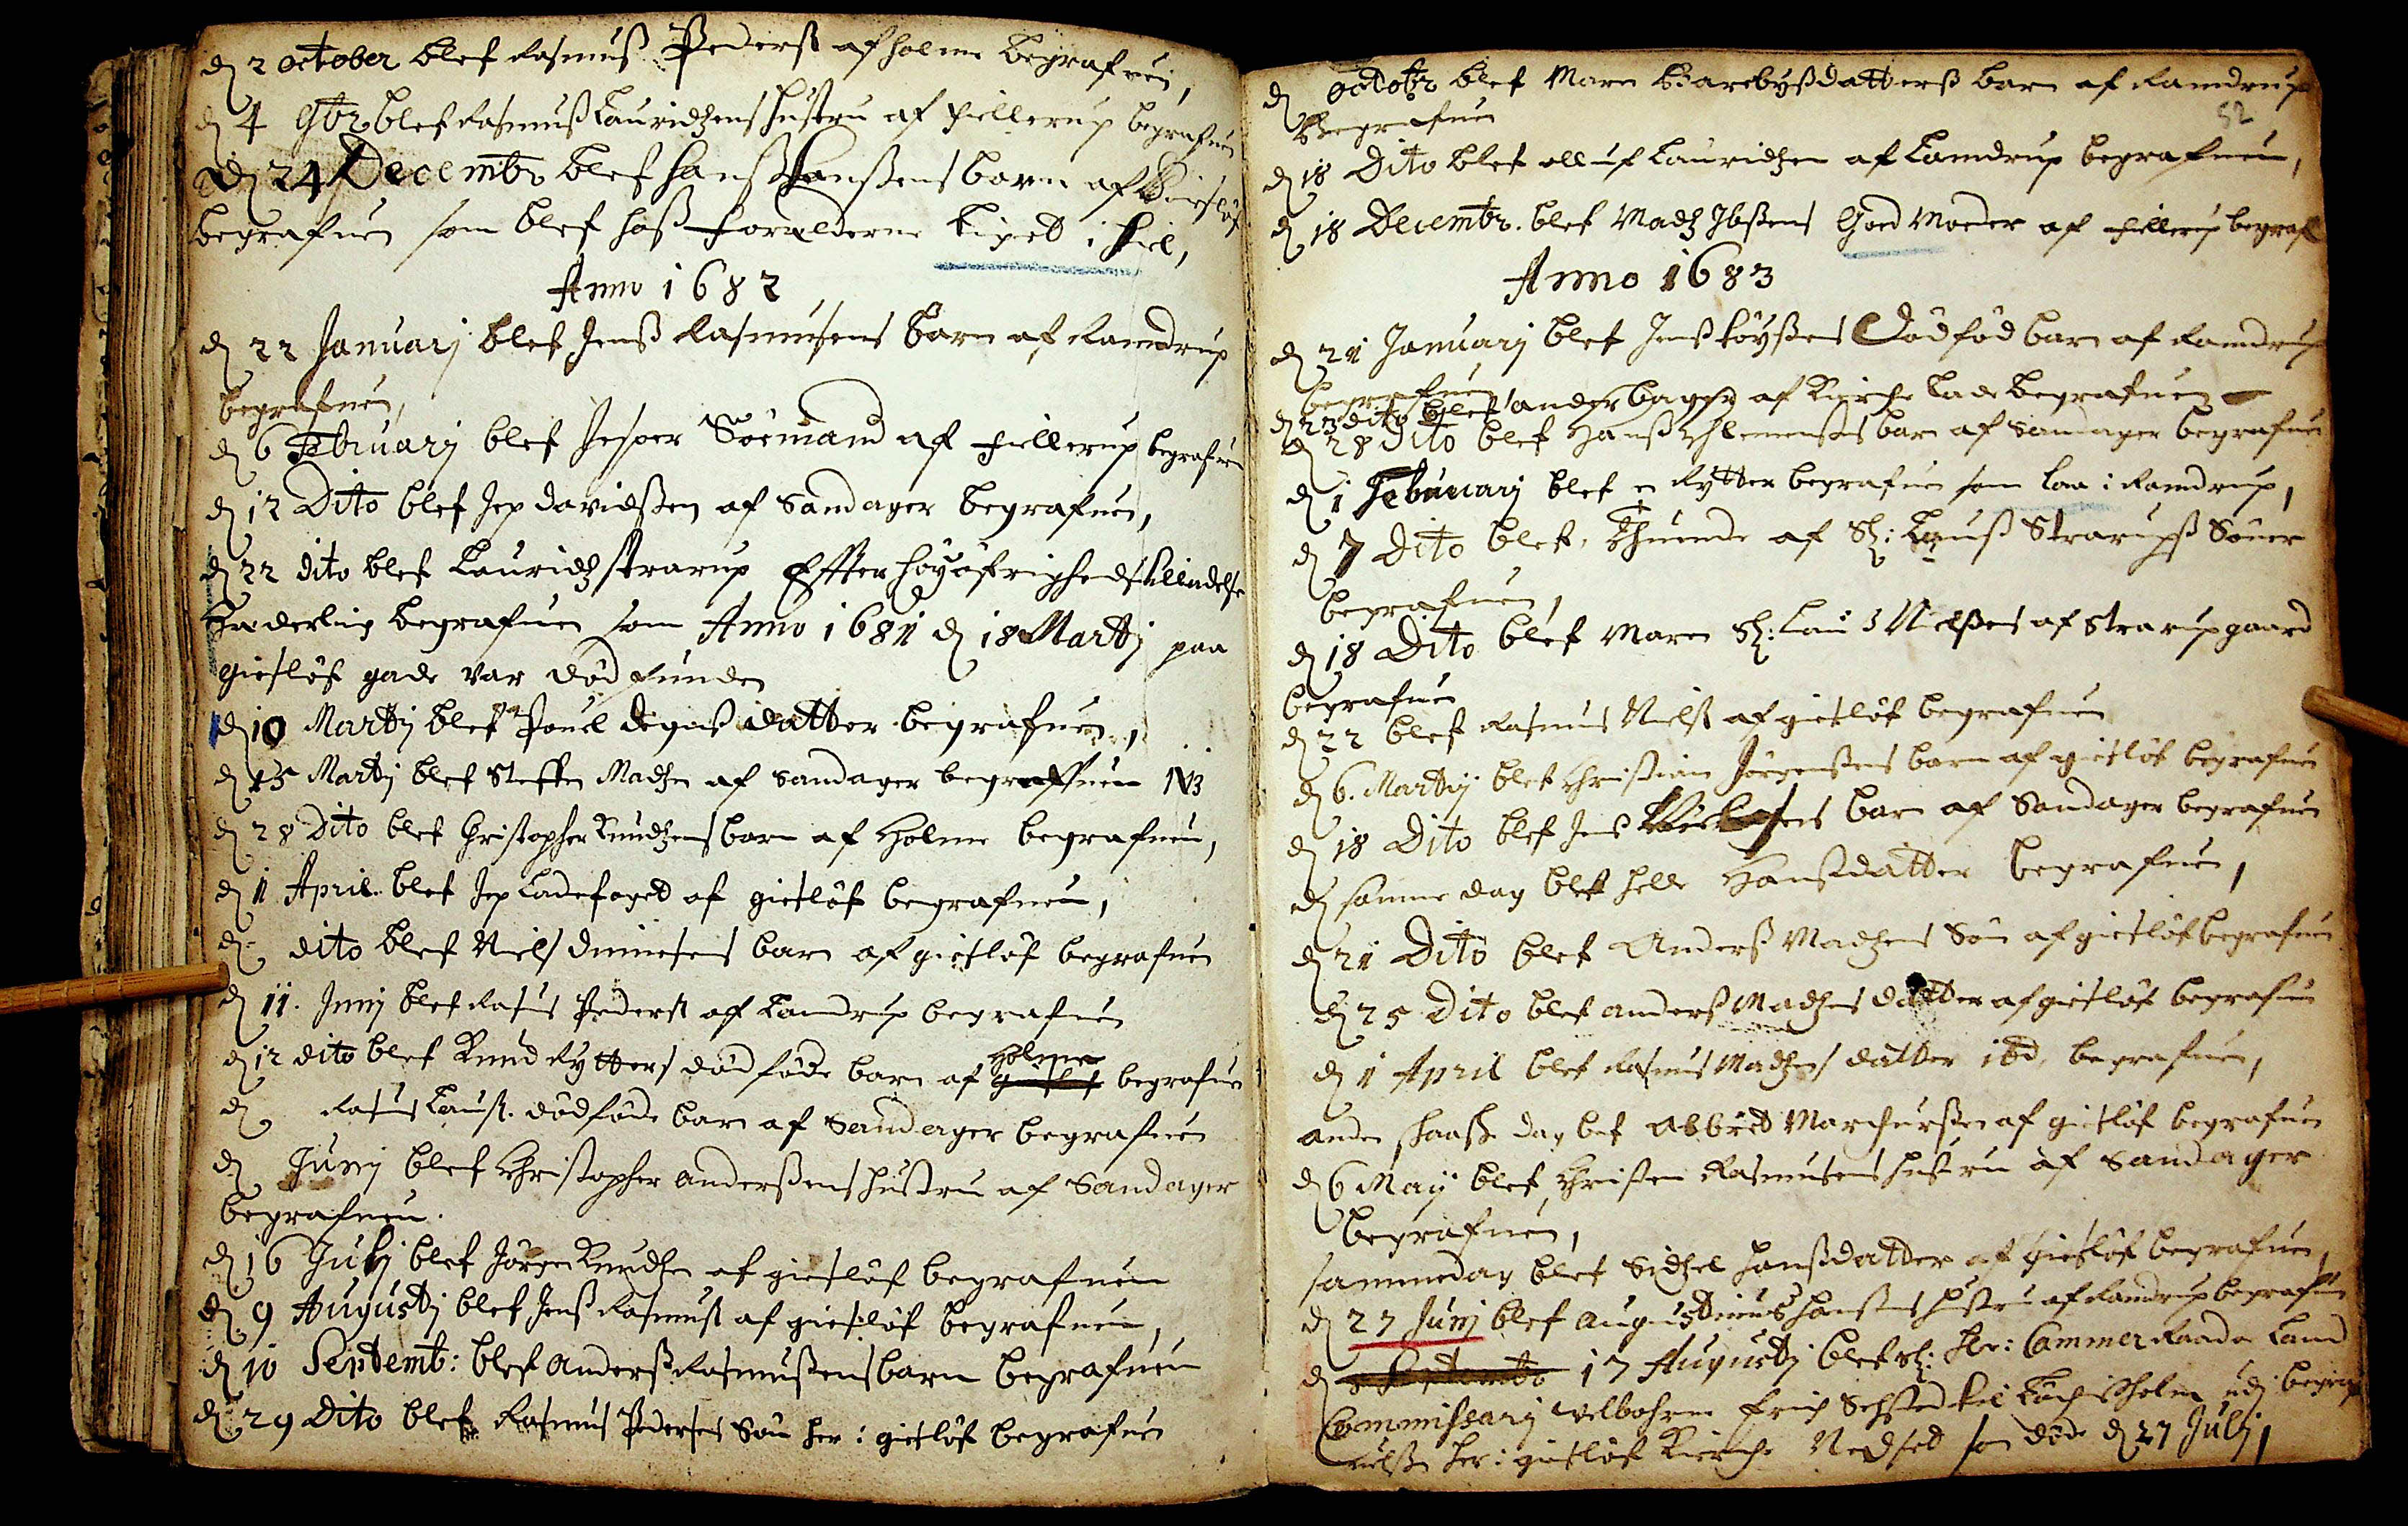d 2 october blef Rasmuss Pederss af holme begraffven
d 4 9br. blef Rasmuss Lauridtzens Hustru af Fiellerup begrafuen
d 24 Decembr blef Hanss Hanssens barn af Giesløf
begrafuen som blef hoss forælderene Liget i Hiel,
Anno 1682
d 22 Januarij blef Jens Rasmusens barn aff RLaredrup
begrafuen
D. 6. Februarij blef Jesper Søemand af Fiellerup begrfuen
d 12 Dito blef Jep Davidssen af Sandager begrafuen,
d 22 dito blef Lauridtz strarup Efter høyofrigheds tilladelse
Hæderlig begrafuen som Anno 1681 d 18 Martij paa
Giesløf gade var død funden
d 10 Martij blef Pouel Degns datter begrafuen
d 25 Martij blef Steffen Madtzen af Sandager begraffuen NB
D 28 Dito blef Christopher Knudtzens barn af Holme begrafuenn
d 1. April blef Jep Lodefoget af Giesløf begraffven
d dito blef Niels Dinneses barn af giesløf begrafuen
d 11. Junij blef Rasmus Peders aff Lamdrup begrafuen
Holme
d 12 dito blef Knud Rytters død føde barn af giesløf begrafuen
d Rasmus Lauss dødføde barn af Sandager begrafuen
d Junij blef Christopher Anderssens hustru af Sandager
begrafuen
d 16 Julij blef Jørgen Knudtzen af giesløf begraffuen
d 9 Augustij blef Jens Rasmuss af giesløf begrafuen
d 10 Septemb: blef Anderss Rasmussens barn begrafuen
d 29 Dito blef Rasmus Pedersens Søn her i Giesløf begrafuen
52
d octobr blef Maren Barebyss datterss barn af Ramderup
Begrafuen
d 18 Dito blef olluf Lauridtzen af Lamdrup begrafuen
d 18 Decembr. blef Madtz Jbssens Gord Moeder af Fiellerup begraf##
Anno 1683
D 21 Januarij blef Jens tøyssens Dødfød barn af Ramdrup
begrafuen
d 23 dito blef ander bager af Kirche Lade begrafuen
d 28 Dito blef Hanss Chlemenssens barn af Sandager begrafuen
d 1 Jebruarij blef en Rytter begrafnen som laa i Ramdrup
d 7 Dito blef Thuinde af Sl: Lauss Strarups Søner
begrafuen
d 18 Dito blef Maren Sl: Lauss Nielssens af Strarupgaard
begrafuen
d 22 blef Rasmus Nielss af giesløf begrafuen
d 6: Martij blef Christian Jørgenssens barn af giesløff begrafuen
d 18 Dito blef Jens Nielsens barn af Sandager begraffuen
d samme dag blef helle Hanssdatter begrafuen,
d 21 Dito blef Anderss Madtzens Søn af giesløf begrafuen
d 25 Dito blef Anderss Madtzens dattter af giesløf begrafuen
d 1 April blef Rasmus Madtzens datter ibd. begrafuen,
anden shaaske dag bef Abbred Marchurssen af giesløf begrafuen
d 6 Maij blef Christen Rasmusens Hustru af Sandager
begrafuen,
sammedag blef Sidtzel Hanssdatter af Giesløf begrafuen
d. 27 Junij blef Augus Dines Hansens hustru af Landrup begraffuen
d 3 Septembr 17 Augustij blef sl: hlr: Cammer Raade Land
Commihsarij Velbohren Erich Sehsted til Løchissholm udj begraf
uelsse her i Giesløf Kierche Ned set som døde d 27 Julij
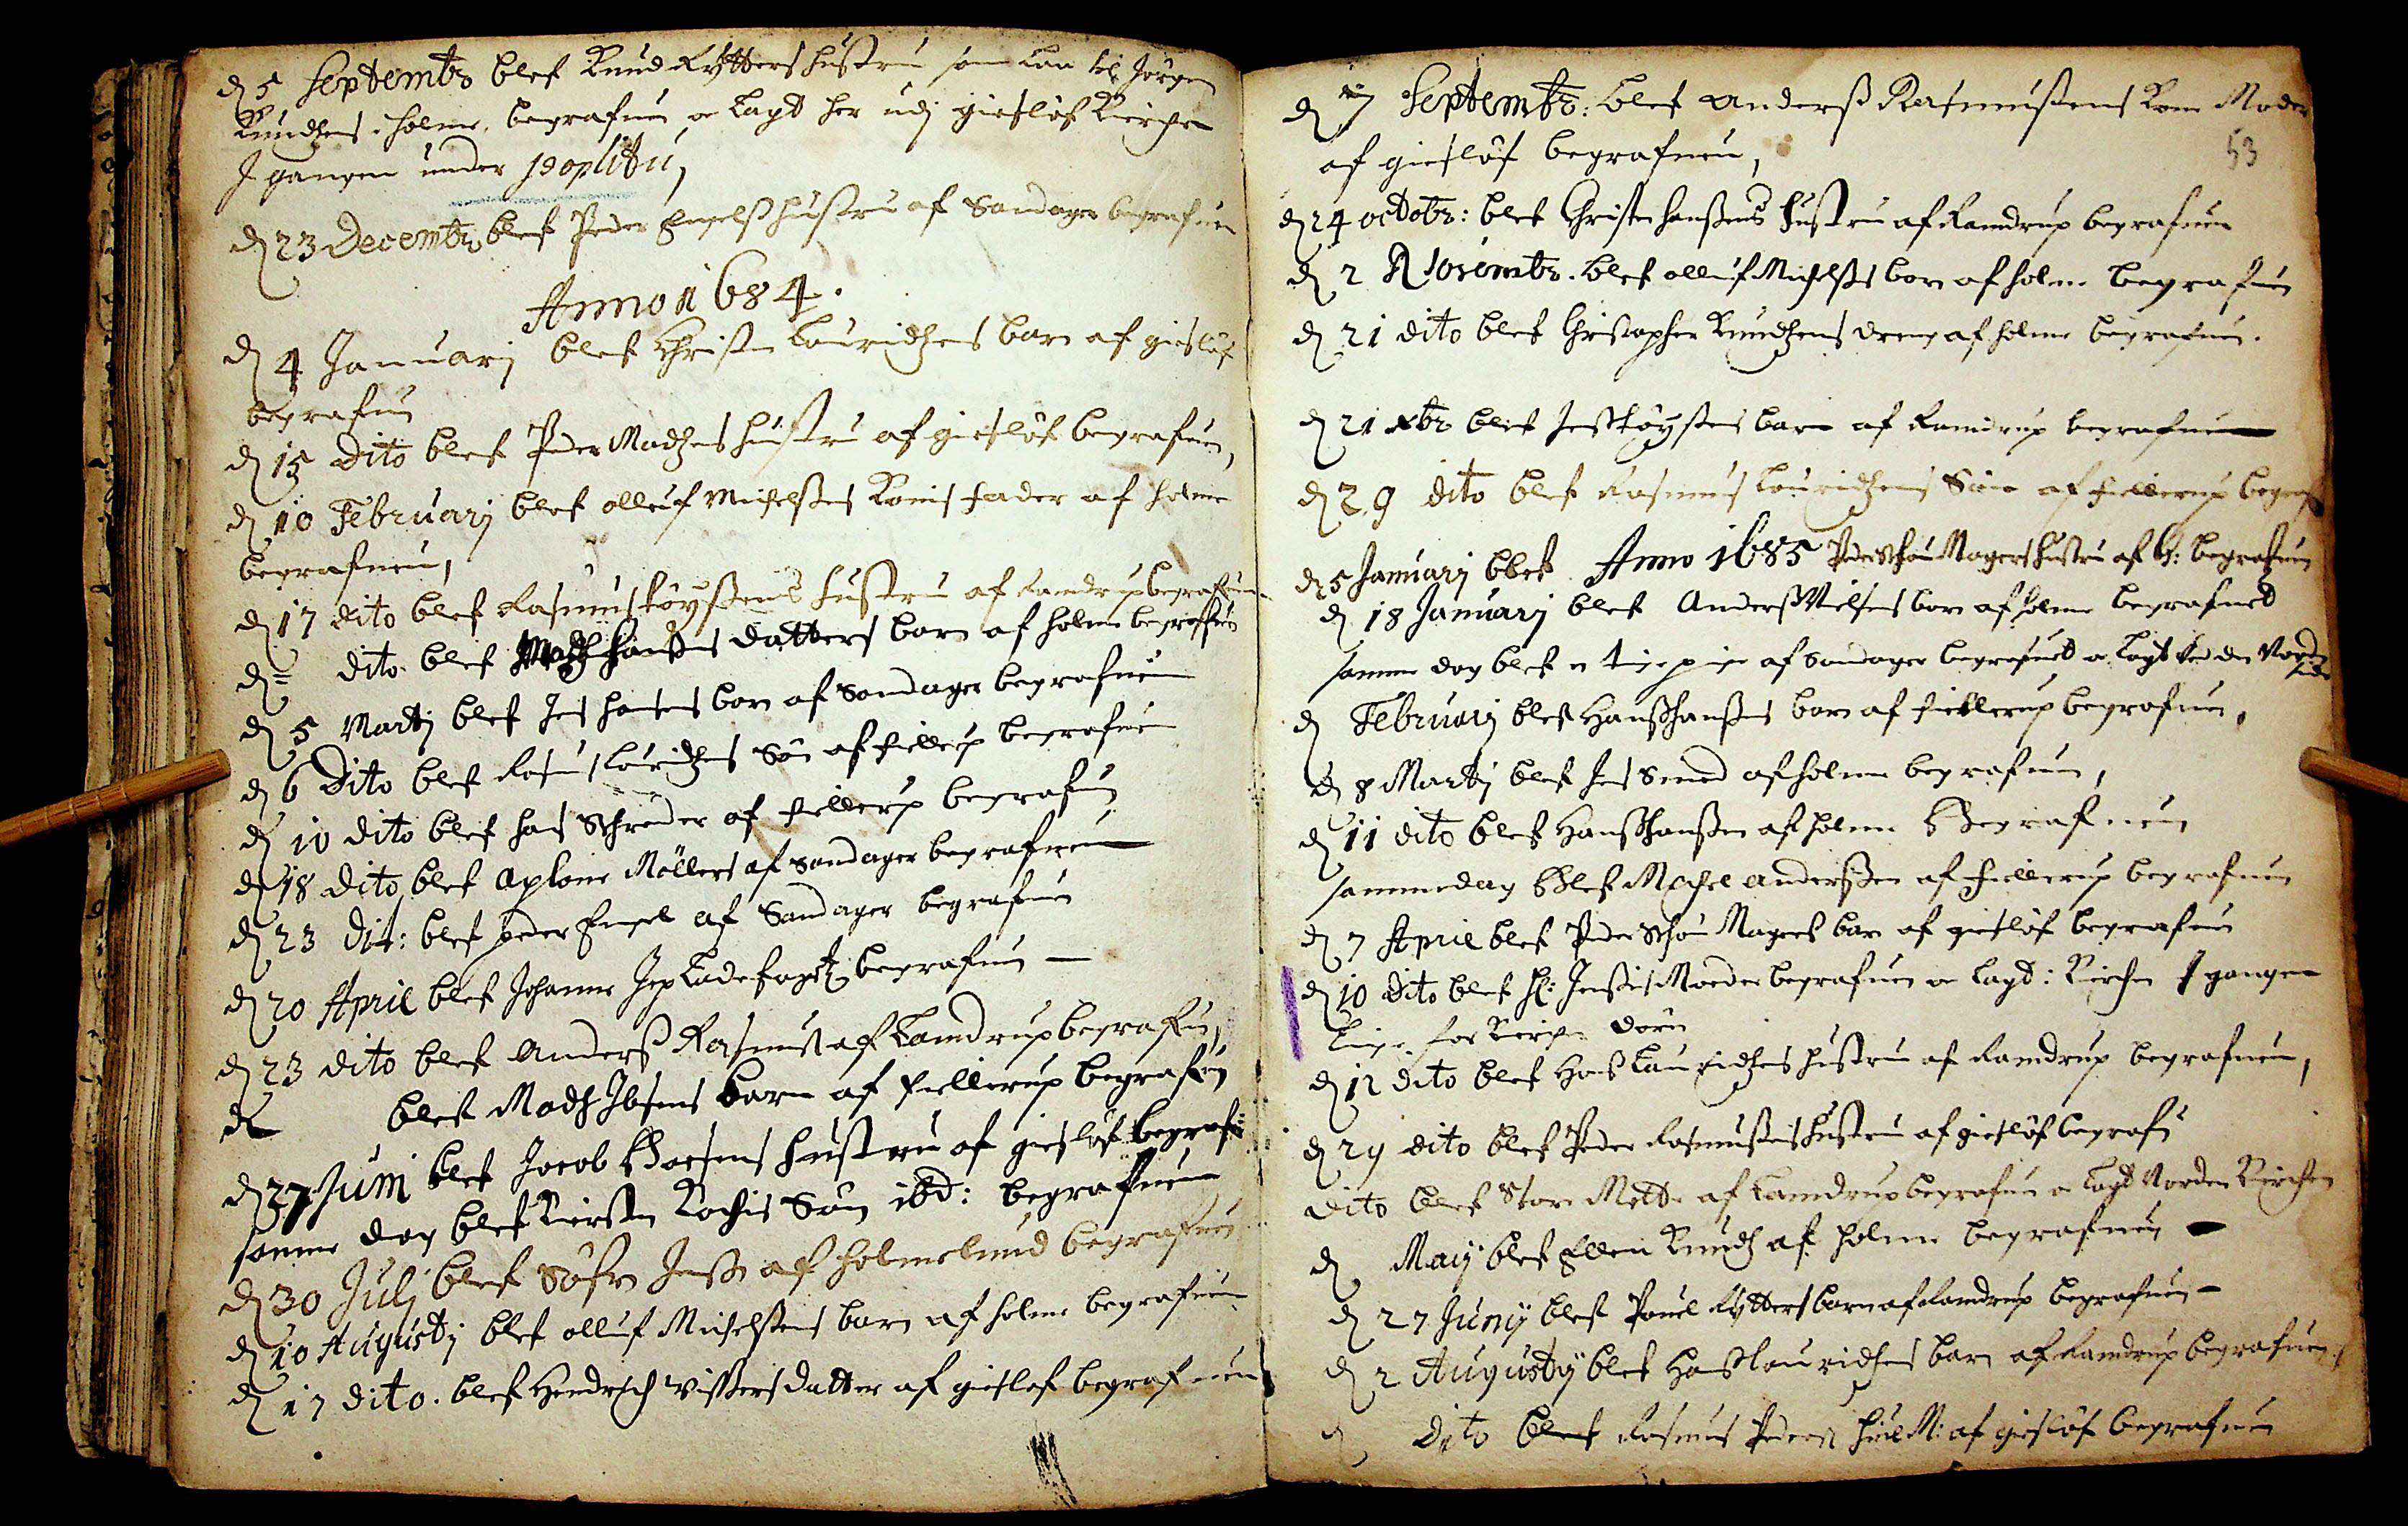d 5 Septembr blef Knud Rytters hustru som laa til Jørgen
Knudtzens i holme begrafuen oc Lagt her udi Giesløf Kierche
I gangen under poplitu,
D 23 Decembr blef Peder Engelss Hustru af Sandager begrafuen
Anno 1684.
d 4 Januarij blef Christen Lauridtzes barn af Giesløf
begrafuen
d 15 Dito blef Peder Madtzens Hustru af Giesløf begrafuen
d 10 Februarj blef Olluf Michelssens Konis Fader af holme
begrafuen
d. 17 Dito blef Rasmus tøyssens Hustru af Lamdrup begrafuen
d= dito. blef Madtz Hanssens datters barn af holm begraffuen
d 5 Martij blef Jens Hansens barn af Sandager begrafuen
d 6 Dito blef Rasmus Lauridtzens Søn af fiellerup begrafuen
d 10 dito blef Hans Schreder af fiellerup begrafuen
d 18 dito blef Aplone Møllers af Sandager begraffuen
d 23. dit: blef Peder Engel af Sandager begrafuen
d 20 April bleff Johanne Jep Ladefogetz begrafuen-
d 23 dito blef Anderss Rasmuss af Lamdrup begraffuen
d
blef Mads Jacob Ibsens barn af Fiellerup begrafuen
d 27 Juni blef Jacob Boesens hustru af giesløf begraf:
samme dag blef Kirsten Kochis Søn ibd: begrafuen
d 30 Julj blef Søfren Jenss af Holmelundbegrafuen
d 10 Augustij blef olluf Michelssens barn af holme begrafuen
d 17 dito. blef Hendeich Vistssens datter af giesløf begraffuen
53
d 7 Septembr: blef Anders Rasmussens Kone Moder
af giesløs begrafuen
d 24 Octobr: blef Christen Hansens Hustru aff Ramdrup begrafuen
d 2 Novembr. blef olluf Michelsens barn af holme begrafuen
d 21 dito blef Christopher Knudtzens dreng af holme begrafuen.
d 21 Xbr blef Jens tøystens barn af Ramdrup begrafuen
d 29 dito blef Rasmus Lauridtzens Søn af Fiellerup begraf
d 5 Januarij bleff Anno 1685 Peder schou Magers Hustru af H. begrafuen
d. 18 Januarij blef Anderss Nelsens barn af holme begrafuet
samme dag blef en t##pige af Sandager begrafuet oc Lagt ved den nordre
side
d Februarij blef Hanss hanssens barn af Fiellerup begrafuen.
d 8 Martij blef Jens Smed of holme begrafuen
d 11 dito blef Hanss Hanssen af holme Begrafuen
sammedag Blef Mechel Anderssen af Fiellerup begrafuen
d 7 April blef Peder Schou Magerss barn af giesløf begrafuen
d 10 Dito blef Hl. Jensis Moeder begrafuen oc Lagt i Kierchen J  gangen
lige for kierche døren
d 12 dito blef Hanss Lauridtzens hustru af Ramdrup begrafuen,
d 29 dito blef Peder Rasmussens Hustru af giesløf begraf##
dito blef Store Mette af Lamdrup begrafuen oc lagt norden Kirchen
d Maij blef Ellen Knudtz af holme begrafuen
d 27 Junij bleff Pouel Rytters barn af Ramdrup begrafuet
d 2 Augustij blef Haslauridzes barn af Lamdrup begrafuen,
d Dito blef Rasmus Pederss hiul M: af giesløf begraffuen